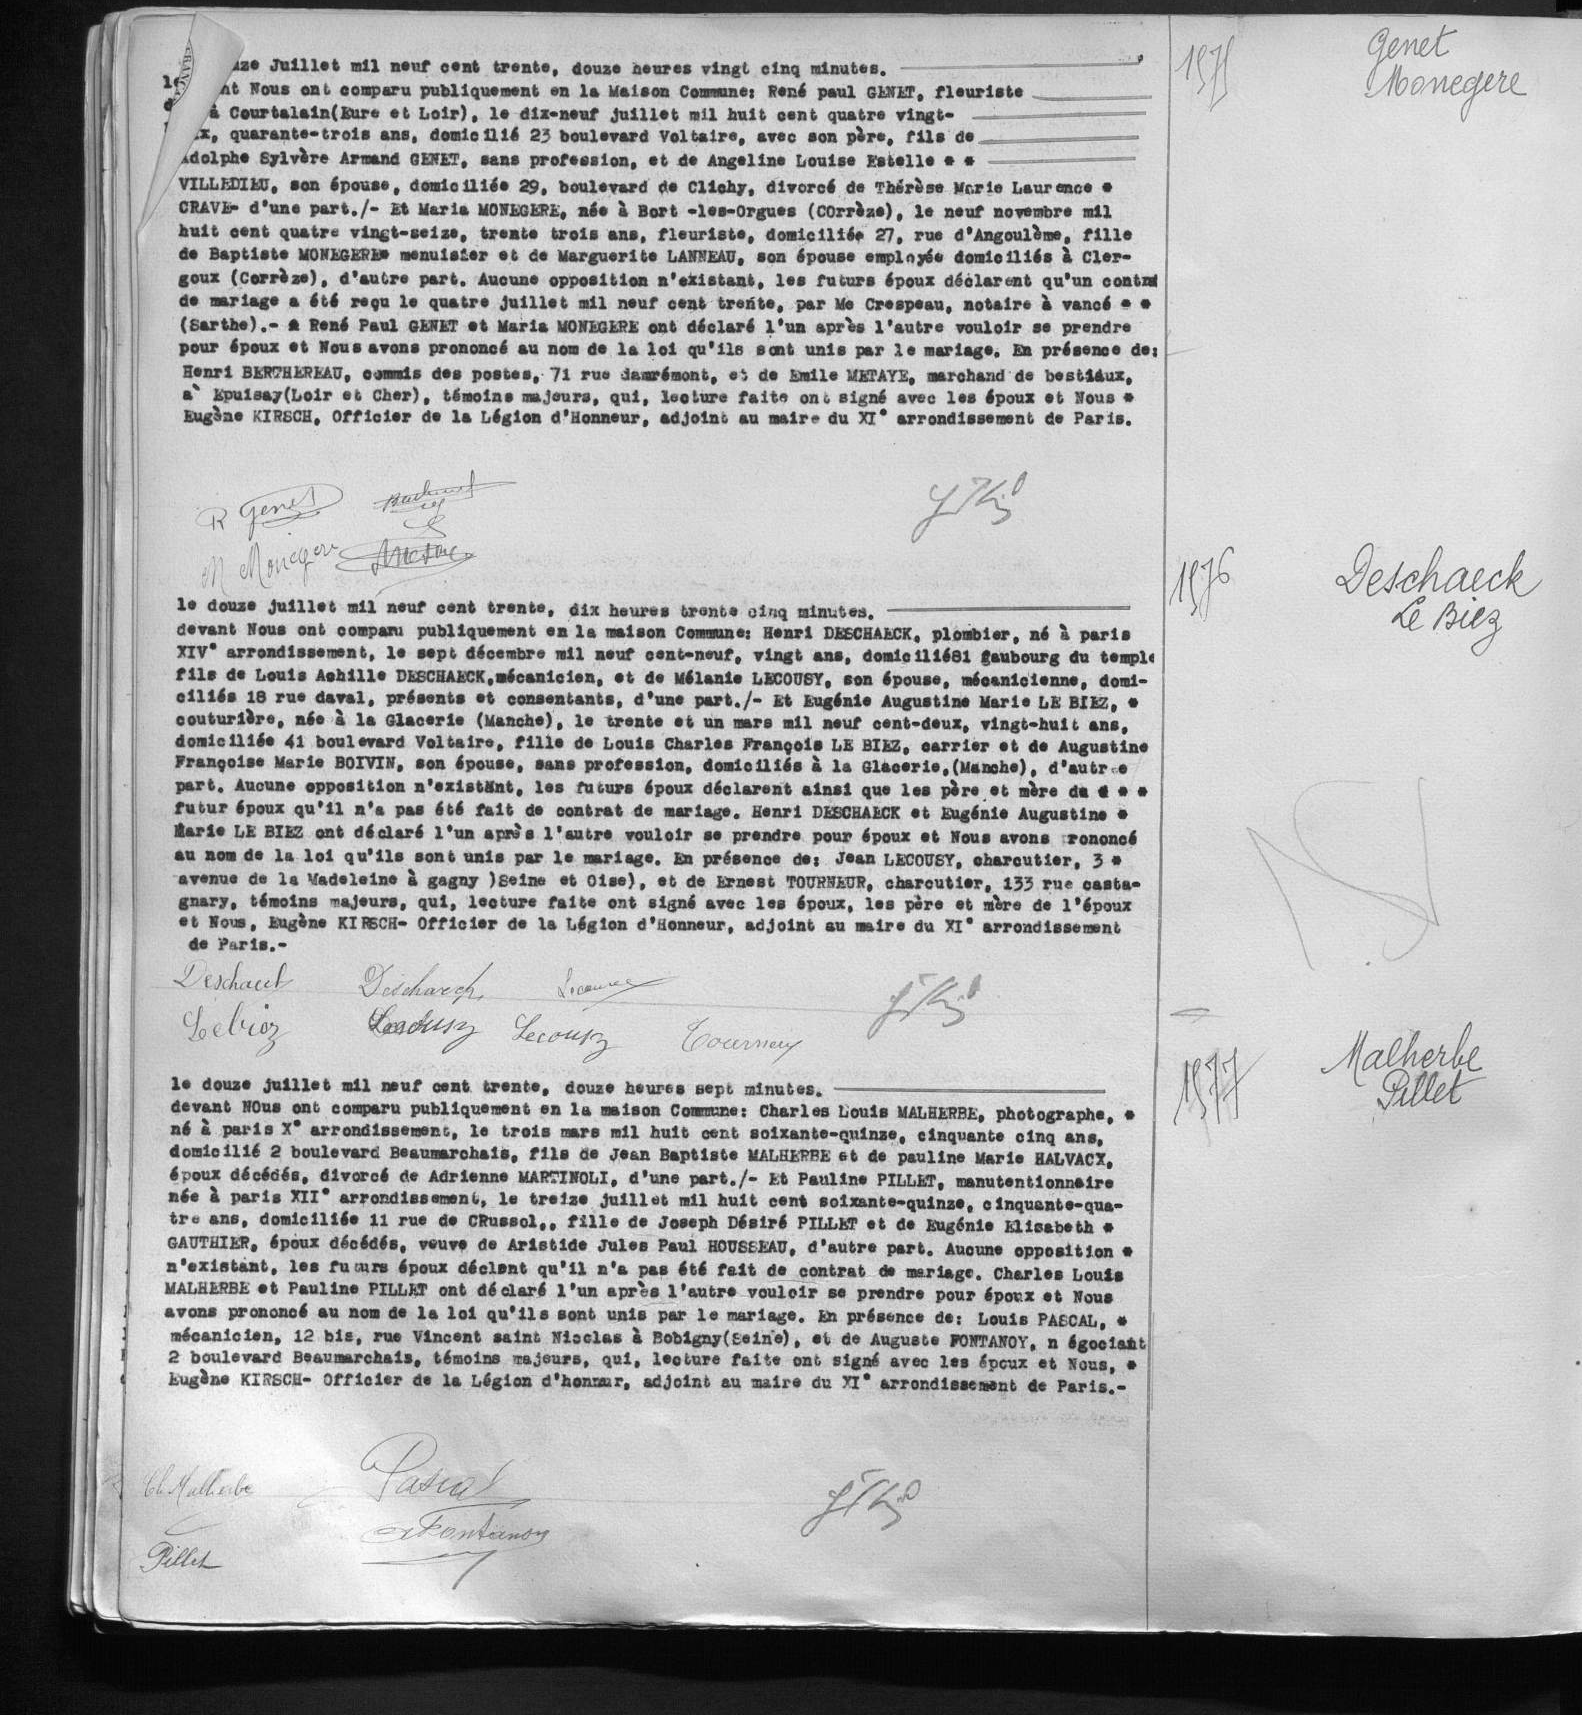uze Juillet mil neuf cent trente, douze heures vingt cinq minutes.-
nt Nous ont comparu publiquement en la Maison Commune: René paul GENET, fleuriste-
à Courtalain (Eure et Loir), le dix-neuf juillet mil huit cent quatre vingt-
x, quarante-trois ans, domicilié 23 boulevard Voltaire, avec son père, fils de-
dolphe Sylvère Armand GENET, sans profession, et de Angeline Louise Estelle ** -
VILLEDIEU, son épouse, domicilié 29, boulevard de Clichy, divorcé de Thérèse Marie Lourence *
CRAVE-d'une part ./-Et Marie MONEGERE, née à Bort-les-Orgues (Corrèze), le neuf novembre mil
huit cent quatre vingt-seize, trente trois ans, fleuriste, domiciliée 27, rue d'Angoulème, fille
de Baptiste MONEGERE * menuisier et de Marguerite LANNEAU, son épouse employée domiciliés à Cler-
goux (Corrèze), d'autre part. Aucune opposition n'existant, les futurs époux déclarent qu'un contra
de mariage a été reçu le quatre juillet mil neuf cent trente, par Me Crespeau, notaire à vancé **
(Sarthe) .-* René Paul GENET et Maria MONEGERE ont déclaré l'un après l'autre vouloir se prendre
pour époux et Nous avons prononcé au nom de la loi qu'ils sont unis par le mariage. En présence de:
Henri BERTHEREAU, commis des postes, 71 rue damrémont, et de Emile METAYE, marchand de bestiaux,
à Epuisay (Loir et Cher), témoins majeurs, qui, lecture faite ont signé avec les époux et Nous *
Eugène KIRSCH, Officier de la Légion d'Honneur, adjoint au maire du XI ° arrondissement de Paris.
le douze juillet mil neuf cent trente, dix heures trente cinq minute.-
devant Nous ont comparu publiquement en la maison Commune: Henri DESCHAECK, plombier, né à paris
XIV ° arrondissement, le sept décembre mil neuf cent-neuf, vingt ans, domicilié 81 faubourg du temple
fils de Louis Achille DESCHAECK, mécanicien, et de Mélanie LECOUSY, son épouse, mécanicienne, domi-
ciliés 18 rue daval, présents et consentants, d'une part ./-Et Eugénie Augustine Marie LE BIEZ ,*
couturière, née à la Glacerie (Manche), le trente et un mars mil neuf cent-deux, vingt-huit ans,
domiciliée 41 boulevard Voltaire, fille de Louis Charles François LE BIEZ, carrier et de Augustine
Françoise Marie BOIVIN, son épouse, sans profession, domiciliés à la Glacerie, (Macnhe), d'autr e
part. Aucune opposition n'existant, les futurs époux déclarent ainsi que les père et mère du ***
futur époux qu'il n'a pas été fait de contrat de mariage. Henri DESCHAECK et Eugénie Augustine *
au nom de la loi qu'ils sont unis par le mariage. En présence de: Jean LECOUSY, charcutier, 3*
avenue de la Madeleine à gagny) Seine et Oise), et de Ernest TOURNEUR, charcutier, 133 rue casta-
gnary, témoins majeurs, qui, lecture faite ont signé avec les époux, les père et mère de l'é poux
et Nous, Eugène KIRSCH-Officier de la Légion d'Honneur, adjoint au maire du XI ° arrondissement
de Paris.-
le douze juillet mil huit cent trente, douze heures sept minutes.-
devant NOus ont comparu publiquement en la maison Commune: Charles Louis MALHERBE, photogaphe, *
né à paris X ° arrondissement, le trois mars mil huit cent soixante-quinze, cinquante cinq ans,
domicilié 2 boulevard Beaumarchais, fils de Jean Baptiste MALHERBE et de pauline Marie HALVACX,
époux décédés, divorcé de Adrienne MARTINOLI, d'une part ./-Et Pauline PILLET, manutentionnaire
née à paris XII ° arrondissement, le treize juillet mil huit cent soixante-quinze, cinquante-qua-
tre ans, domiciliée 11 rue de CRussol ,, fille de Josephe Désiré PILLET et de Eugénie Elisabeth *
GAUTHIER, époux décédés, veuve de Aristide Jules Paul HOUSSEAU, d'autre part. Aucune opposition *
n'existant, les futurs époux déclent qu'il n'a pas été fait de contrat de mariage. Charles Louis
MALHERBE et Pauline PILLET ont déclaré l'un après l'autre vouloir se prendre pour époux et Nous
avons prononcé au nom de la loi qu'ils sont unis par le mariage. En présence de: Louis PASCAL, *
mécanicien, 12 bis, rue Vincent saint Nicolas à Bobigny (Seine), et de Auguste FONTANOY, n égociant
2 boulevard Beaumarchais, témoins majeurs, qui, lecture faite ont signé avec les époux et Nous, *
Eugène KIRSCH-Officier de la Légion d'honneur, adjoint au maire du XI ° arrondissement de Paris.-
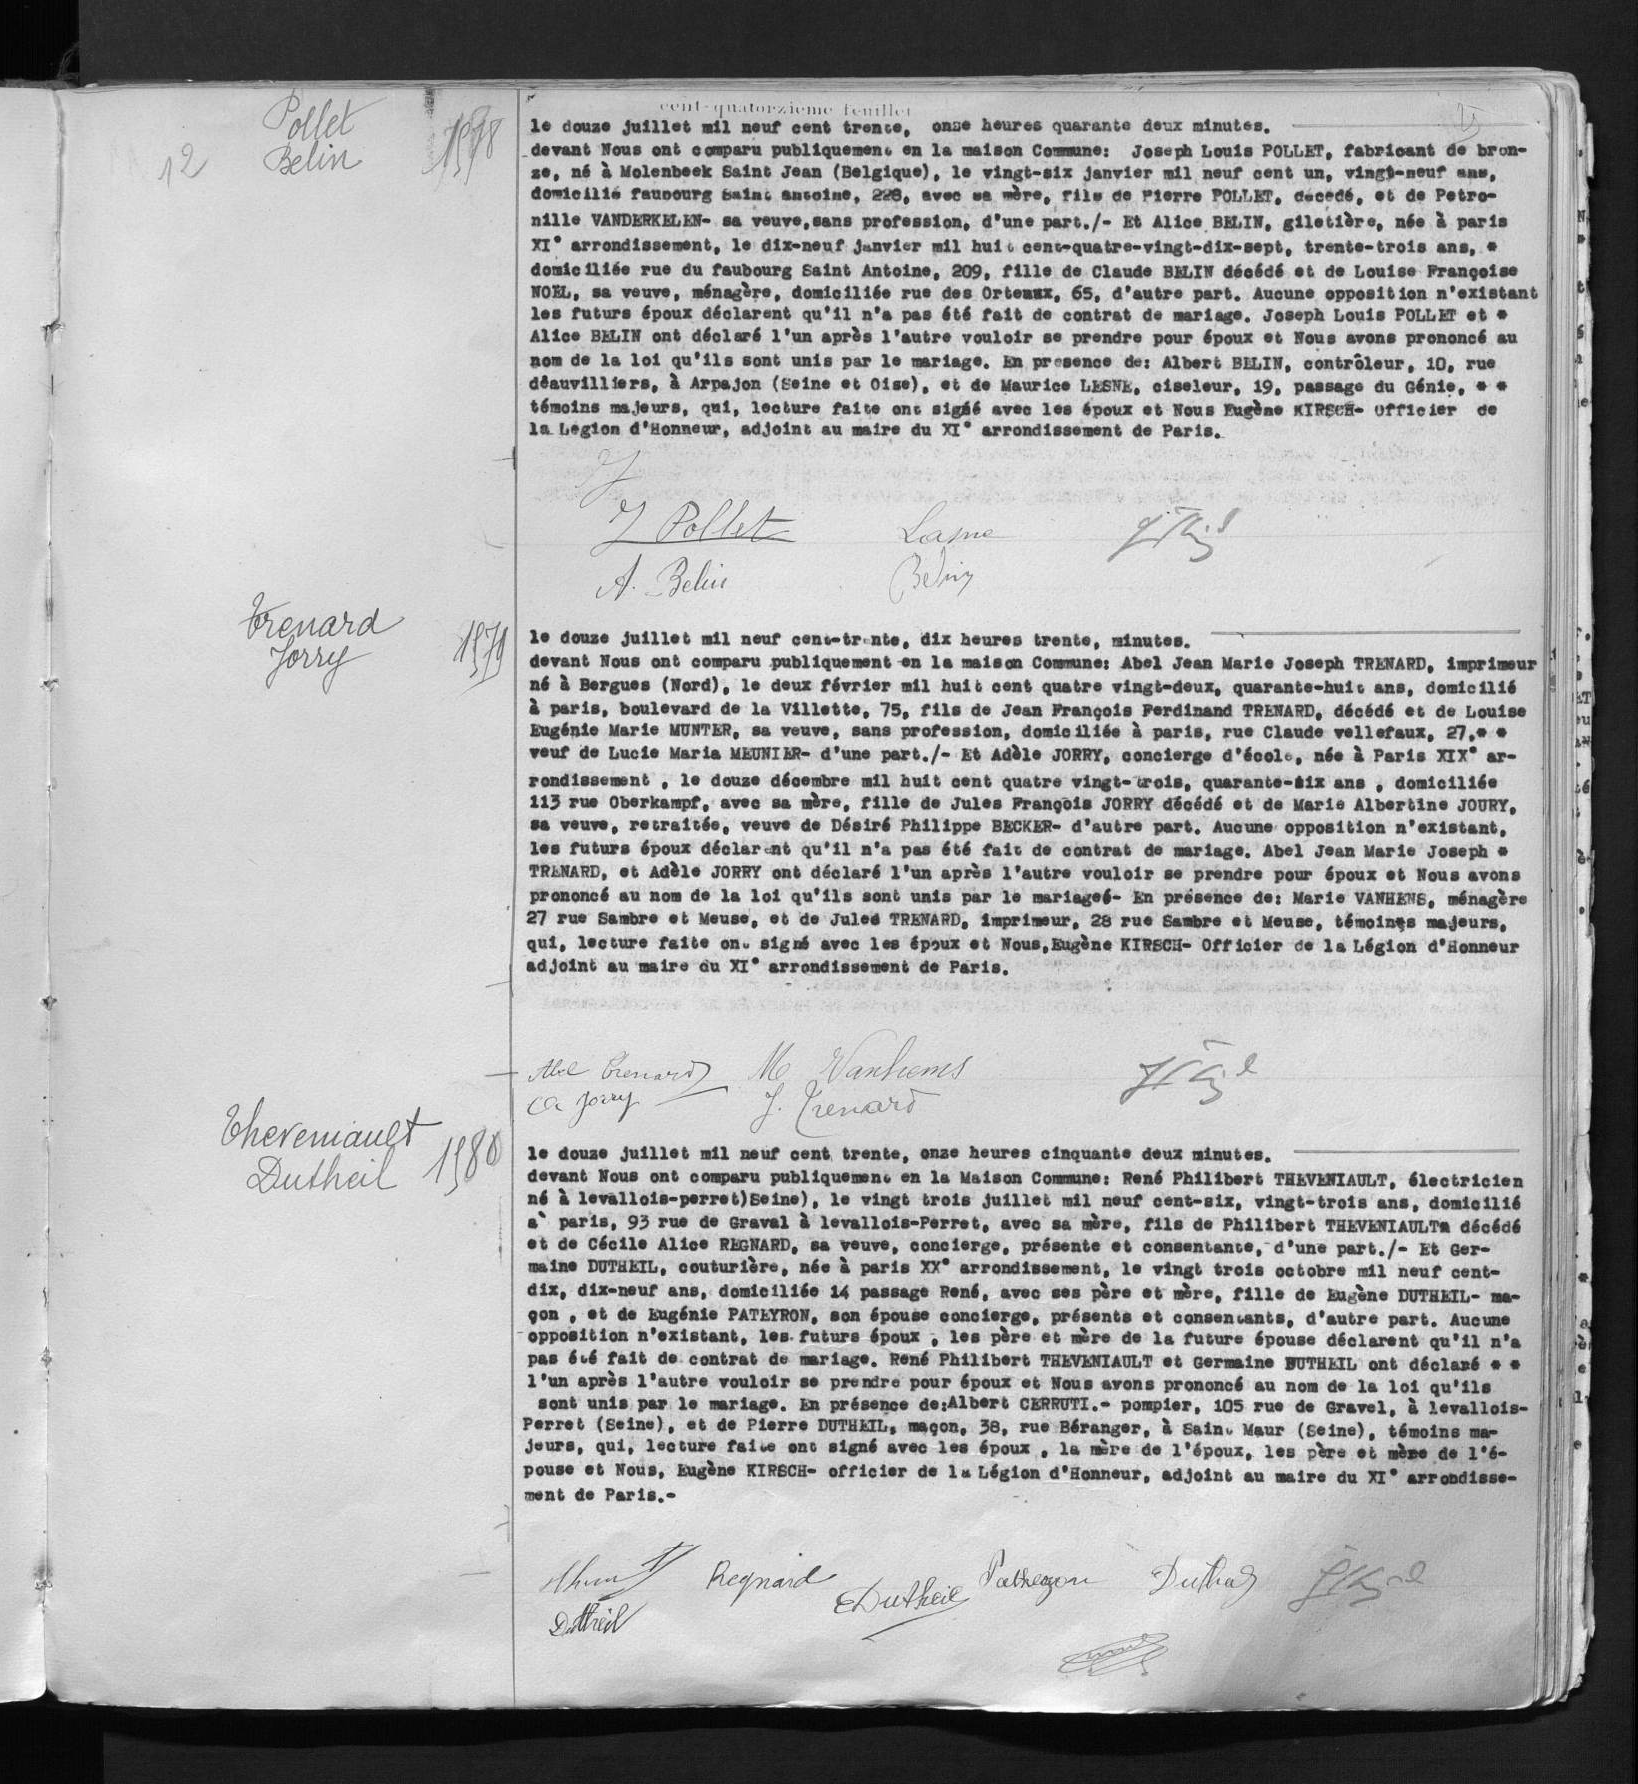le douze juillet mil neuf cent trente, onze heures quarante deux minutes. -
devant Nous ont comparu publiquement en la maison Commune: Joseph Louis POLLET, fabricant de bron-
ze, né à Molenbeek Saint Jean (Belgique), le vingt-six janvier mil neuf cent un, vingt-neuf ans,
domicilié faubourg Saint antoine, 228, avec sa mère, fils de Pierre POLLET, décédé, et de Petro-
nille VANDERKELEN-sa veuve, sans profession, d'une part ./-Et Alice BELIN, giletière, née à paris
XI ° arrondissement, le dix-neuf janvier mil huit cent-quatre-vingt-dix-sept, trente-trois ans, *
domiciliée rue du faubourg Saint Antoine, 209, fille de Claude BELIN décédé et de Louise Françoise
NOEL, sa veuve, ménagère, domiciliée rue de Orteaux, 65, d'autre part. Aucune opposition n'existant
les futurs époux déclarent qu'il n'a pas été fait de contrat de mariage. Joseph Louis POLLET et *
Alice BELIN ont déclaré l'un après l'autre vouloir se prendre pour époux et Nous avons prononcé au
nom de la loi qu'ils sont unis par le mariage. En presence de: Albert BELIN, contrôleur, 10, rue
deauvilliers, à Arpajon (Seine et Oise), et de Maurice LESNE, ciseleur, 19, passage du Génie, **
témoins majeurs, qui, lecture faite ont signé avec les époux et Nous Eugène KIRSCH-Officier de
la Legion d'Honneur, adjoint au maire du XI ° arrondissement de Paris.
le douze juillet mil neuf cent-trente, dix heures trente, minutes. -
devant Nous ont comparu publiquement en la maison Commune: Abel Jean Marie Joseph TRENARD, imprimeur
né à Bergues (Nord), le deux février mil huit cent quatre vingt-deux, quarante-huit ans, domicilié
à paris, boulevard de la Villette, 75, fils de Jean François Ferdinand TRENARD, décédé et de Louise
Eugénie Marie MUNTER, sa veuve, sans profession, domiciliée à paris, rue Claude vellefaux, 27, **
veuf de Lucie Maria MEUNIER-d'une part ./-Et Adèle JORRY, concierge d'école, née à Paris XIX ° ar-
rondissement, le douze décembre mil huit cent quatre vingt-trois, quarante-six ans, domiciliée
113 rue Oberkampf, avec sa mère, fille de Jules François JORRY décédé et de Marie Albertine JOURY,
sa veuve, retraitée, veuve de Désiré Philippe BECKER-d'autre part. Aucune opposition n'existant,
les futurs époux déclarent qu'il n'a pas été fait de contrat de mariage. Abel Jean Marie Joseph *
TRENARD et Adèle JORRY ont déclaré l'un après l'autre vouloir se prendre pour époux et Nous avons
prononcé au nom de la loi qu'ils sont unis par le mariageé-En présence de: Marie VANHENS, ménagère
27 rue Sambre et Meuse, et de Jules TRENARD, imprimeur, 28 rue Sambre et Meuse, témoins majeurs,
qui, lecture faite ont signé avec les époux et Nous, Eugène KIRSCH-Officier de la Légion d'Honneur
adjoint au maire du XI ° arrondissement de Paris.
le douze juillet mil huit cent trente, onze heures cinquante deux minutes. -
devant Nous ont comparu publiquement en la Maison Commune: René Philibert THEVENIAULT, électricien
né à levallois-perret) Seine), le vingt trois juillet mil neuf cent-six, vingt-trois ans, domicilié
à paris, 93 rue de Graval à levallois-Perret, avec sa mère, fils de Philibert THEVENIAULT décédé
et de Cécile Alice REGNARD, sa veuve, concierge, présente et cosentante, d'une part ./-Et Ger-
maine DUTHEIL, couturière, née à paris XX ° arrondissement, le vingt trois octobre mil neuf cen
dix, dix-neuf ans, domiciliée 14 passage René, avec ses père et mère, fille de Eugène DUTHEI-ma-
çon, et de Eugénie PATEYRON, son épouse concierge, présents et consentants, d'autre part. Aucune
opposition n'existant, les futurs époux, les père et mère de la future épouse déclarent qu'il n'a
pas été fait de contrat de mariage. René Philibert THEVENIAULT et Germaine BUTHEIL ont déclaré **
l'un après l'autre vouloir se prendre pour époux et Nous avons pronocné au nom de la loi qu'ils
sont unis par le mariage. En présence de: Albert CERRUTI .-pompier, 105 rue de Gravel, à levallois-
Perret (Seine), et de Pierre DUTHEIL, maçon, 38, rue Béranger, à Saint Maur (Seine), témoins ma-
jeurs, qui, lecture faite ont signé avec les époux, la mère de l'époux, les père et mère de l'é-
pouse et Nous, Eugène KIRSCH-officier de la Légion d'Honneur, adjoint au maire du XI ° arrondisse-
ment de Paris.-